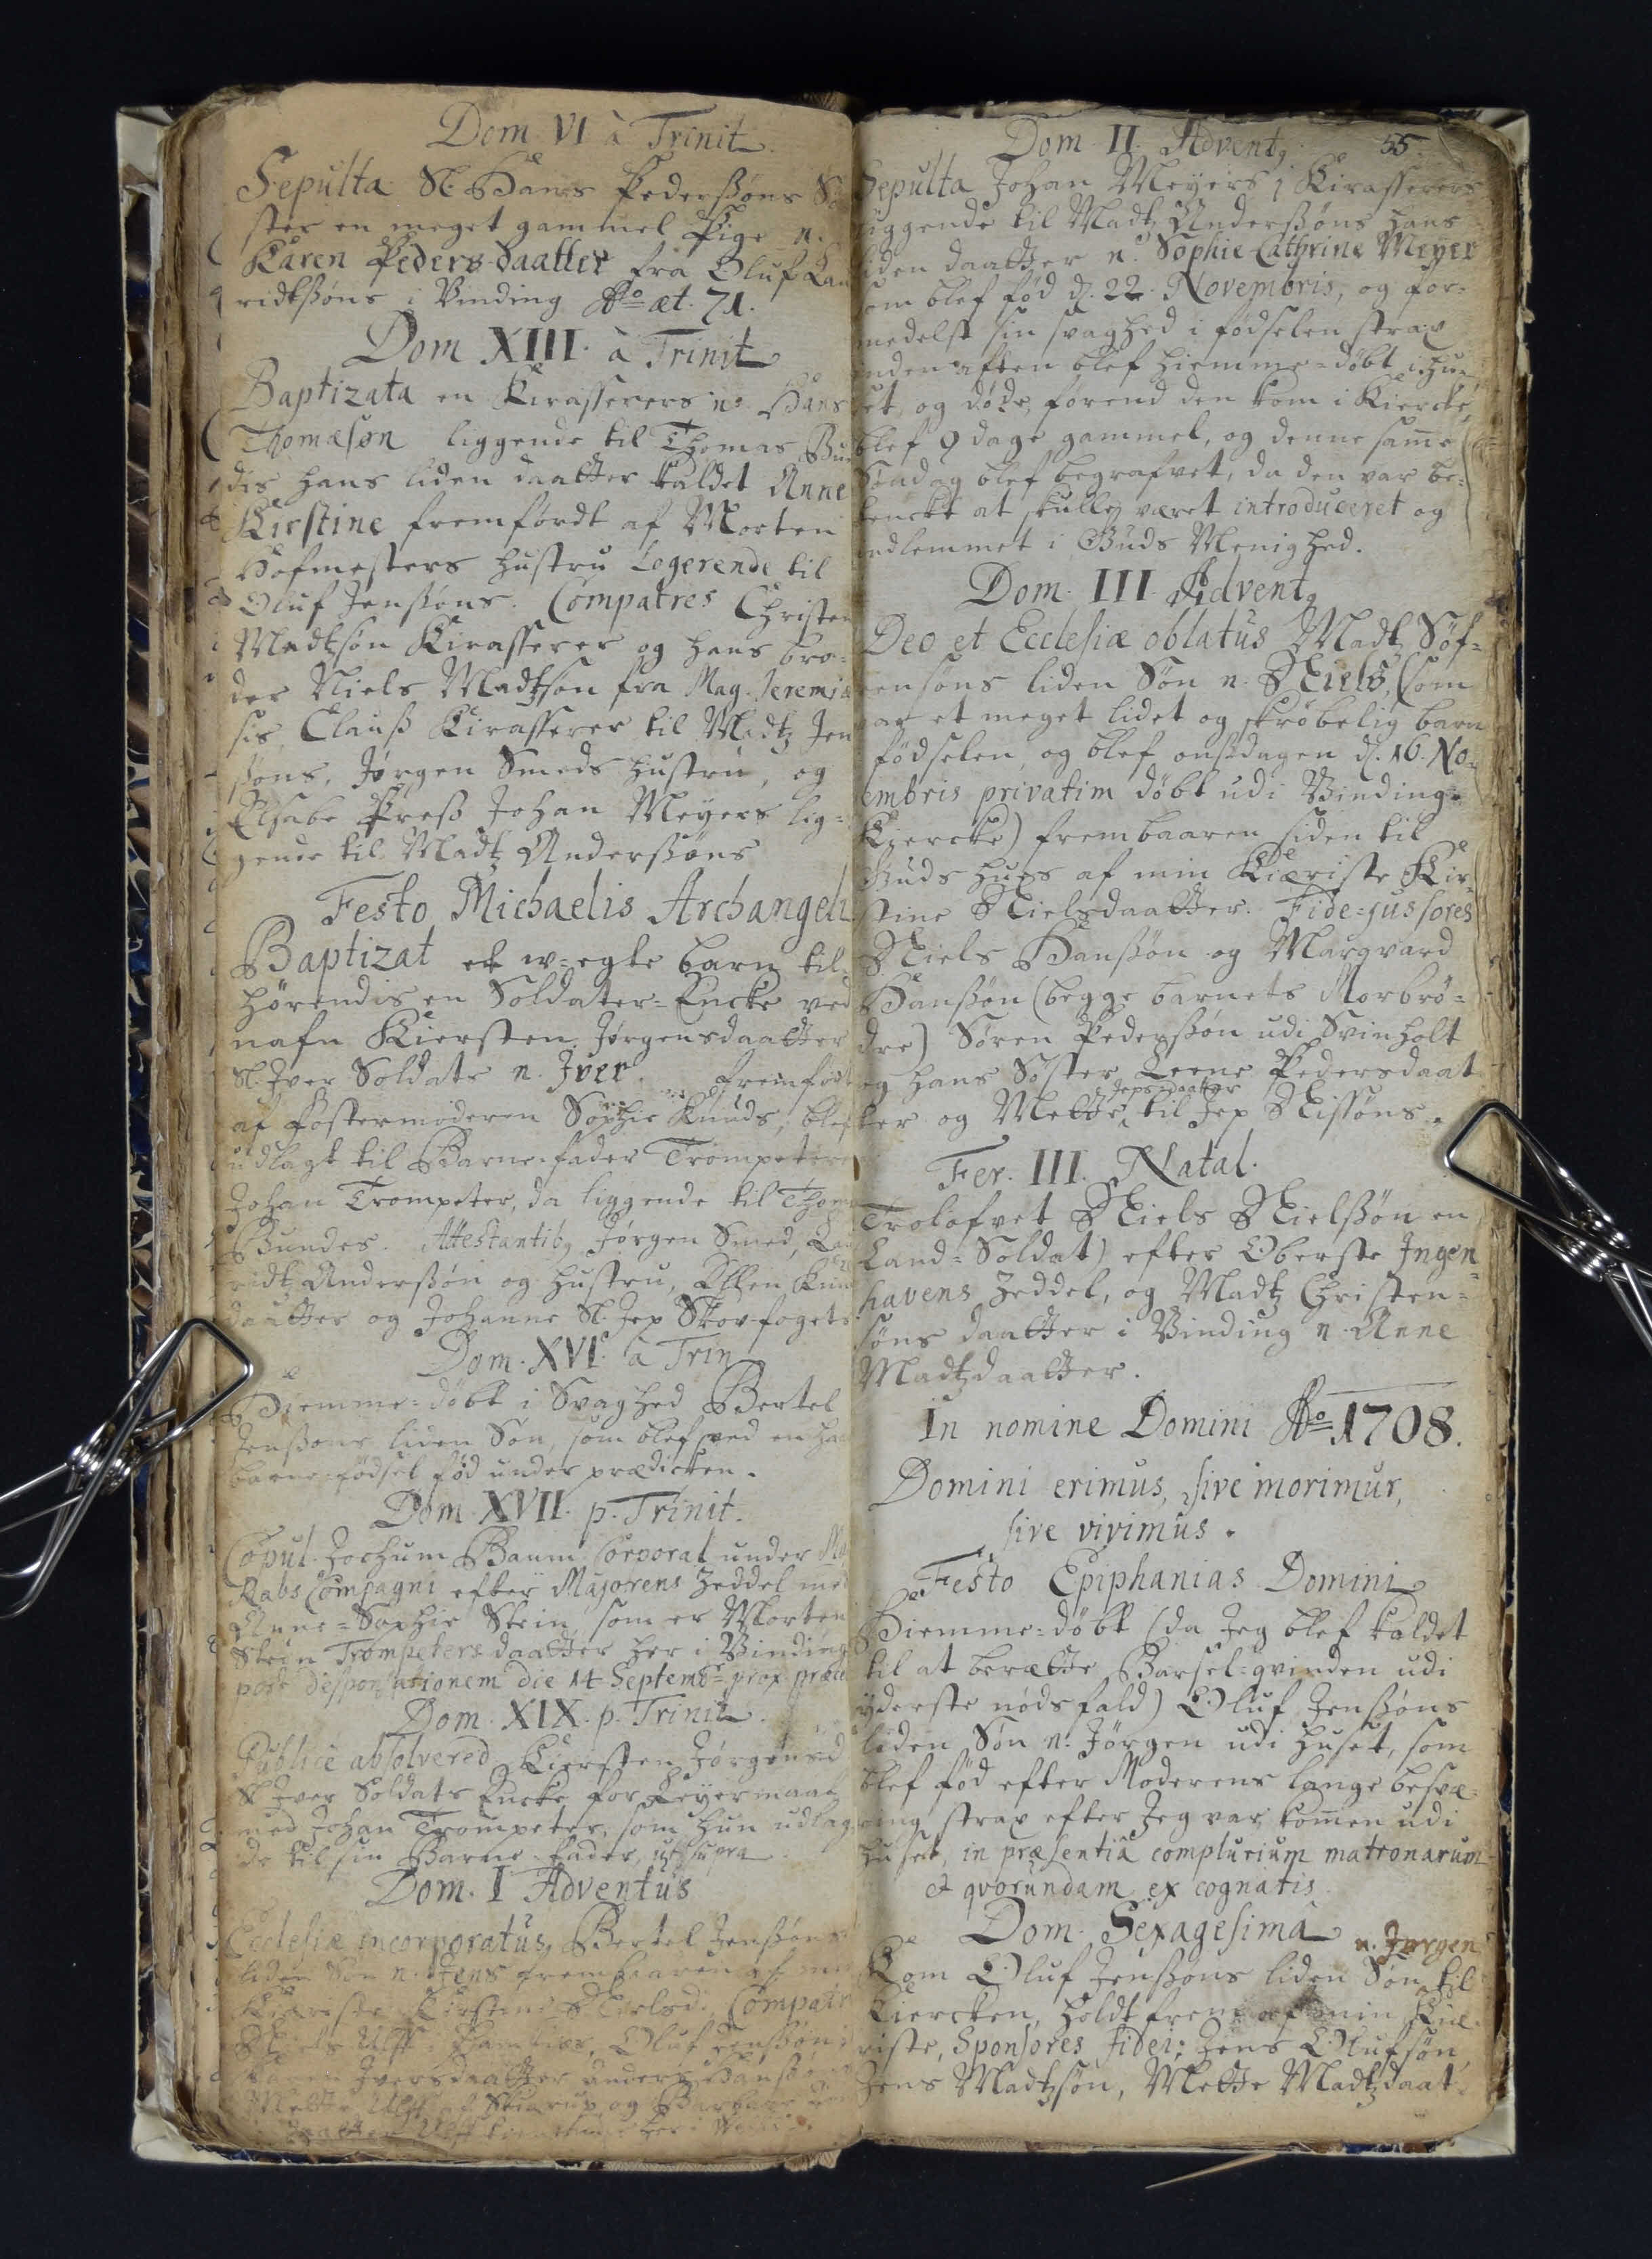Dom. VI a Trinit
Sepulta. Sl. Hans Pederssøns Sø
ster en meget gammel Pige n.
Karen Peders-daatter fra Oluf Lau
ridtssøns i Vinding Ao æt. 71.
Dom XIII. a Trinit
Baptizata en Kirasserer n. Hans
Thomæsøn liggende til Thomas Bun
dis hans liden daatter kaldet Anne
Kirstine fremførdt af Morten
Hofmesters hustru Logerende til
Oluf Jenssøns. Compatres Christen
Madtsøn Kirasserer og hans bro¬
der Niels Madtsøn fra Mag. Jeremiæ
sis, Claus Kirasserer til Madtz Jen
ssøns, Jørgen Smeds hustru, og
Elsabe Press Johan Meyers lig¬
gende til Madtz Anderssøns
Festo Michaelis Archangetz.
Baptizat et w=egte barn til
hørendis en Soldater=Encke ved
nafn Kiersten Jørgensdaatter
Sl. Iver Soldats n. Iver, fremført
af Fostermoderen Sophie Knuds, blef
udlagt til Barne=fader Trompeter
Johan Trompeter, da liggende til
Bundes. Attestantibq Jørgen Smed, Lau
ridtz Anderssøn og hustru, Ellen
daatter og Johanne Sl. Jep Skov-fogets
Dom. XVI a. Trin
Hiemme = døbt i Svaghed Bertel
Jenssøns liden Søn, som blef ved
barne = fødsel fød under prædicken.
Dom. XVII. p. Trinit.
Copul. Jochum Baum Corporal under Ma 
Rabs Compagni efter Majorens Zeddel med
Anne = Sophie Stein, som er Morten
Stein Trompeters daatter her i Vinding
post## Desponsationem die 14 Septemb = prox## præ##
Dom. XIX. p. Trinit.
Publice absolvered Kiersten Jørgensd
Sl. Ivers Soldats Encke for Leyermaal
med Johan Trompeter, som hun udlag¬
de til sin Barne = fader, ut supra.
Dom. I Adventus
Ecclesiæ in corporatus Bertel Jenssøns
liden Søn n. Jens frembaaren af
Kiæriste Kierstine Nielsd. Compatr 
Niels Ulff i Damkier. Oluf Jenssøn.
Iversdaatter Anders Hanssøns
Mette Ulff af Skiærup og Barbara
datter Ulff ## hos i W##
55
Dom. II. Adventq
Sepulta Jørgen Meyers 1 Kirasserers
liggende til Madtz Anderssøns hans
liden daatter n. Sophie Cathrine Meyer
som blef fød d. 22 Novembris, og for¬
medelst sin svaghed i fødselen strax
inden aften blef hiemme=døbt i hu¬
set, og døde førend den kom i Kircke,
blef 9 dage gammel, og denne samme
Søndag blef begrafvet, da den var be¬
tenckt at skulle været introduceret og
indlemmet i Guds Menighed.
Dom. III Adventq
Deo et Ecclesiæ oblatus Madtz Søf¬
rensøns liden Søn n. Niels (som
var et meget lidet og skrøbelig barn
fødselen, og blef onssdagen d. 16 No¬
embris privatim døbt udi Vinding
Kircke) frembaaren siden til
Guds Huus af min Kiæriste Kir¬
stine Nielsdaatter. Fide=jussores
Niels Hanssøn og Marqvard
Hanssøn (begge barnets Morbrø¬
dre) Søren Pederssøn udi Svinholt
og hans Søster Leene Pedersdaat¬
Jepsdaatter
ter og Mette til Jep Nissøns.
Fer. III. Natal.
Trolofvet Niels Nielssøn en
Land = Soldat efter Oberste Ingen¬
havens Zeddel, og Madtz Christen¬
søns daatter i Vinding n. Anne
Madtzdaatter.
In nomune Domini Ao 1708.
Domini erimus, sive morimur,
sive vivimus.
Festo Epiphanias Domini
Hiemme=døbt (da jeg blef kaldet
til at berætte Barsel=qvinden udi
yderste nøds fald) Oluf Jenssøns
liden Søn n. Jørgen udi huset, som
blef fød efter Moderens lange besvæ¬
ring strax efter jeg var komen udi
huset, in præsentia complurium matronarum
et qvorundam ex cognatis
Kom Oluf Jenssøns liden Søn til
Dom. Sexagesima n. Jørgen
n. Jørgen
Kircken, holdt frem af min Kiæ¬
riste. Spopnsores fidei; Jens Olufsøn,
Jens Madtzsøn, Mette Madtzdaat¬
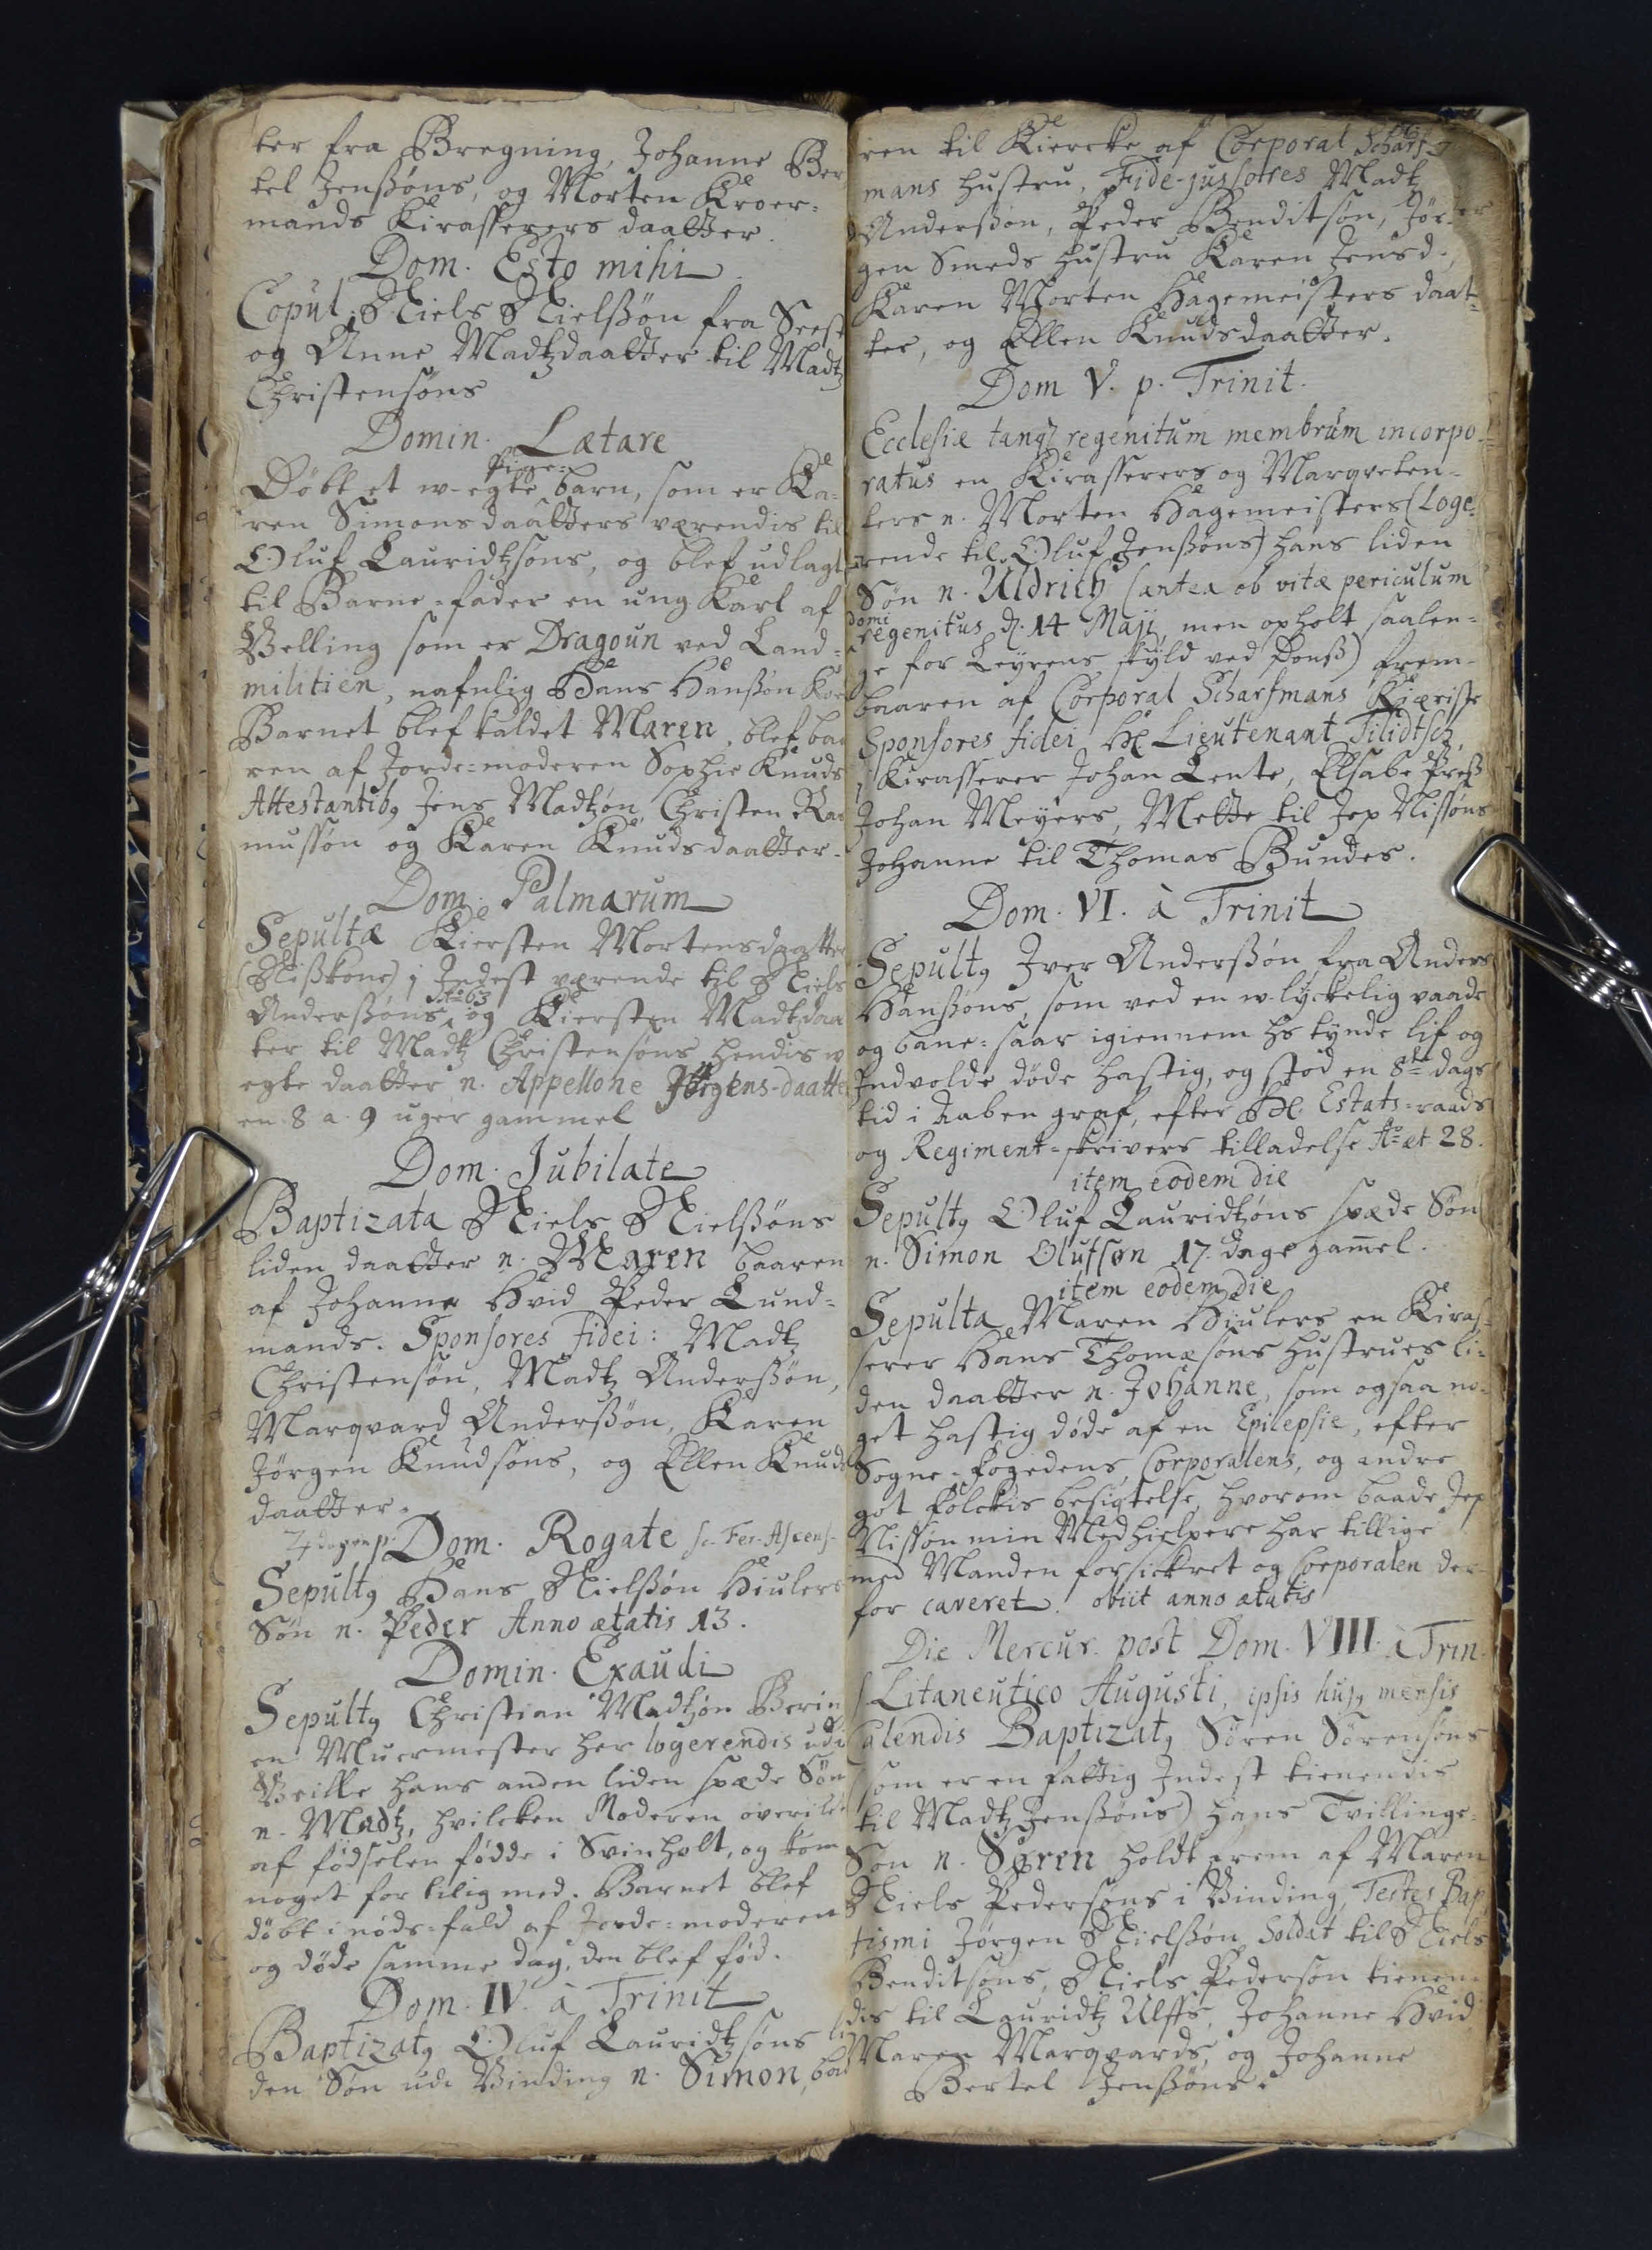ter fra Breining, Johanne Ber¬
tel Jenssøns, og Morten Kroer¬
mands Kirasserers daatter
Dom. Esto mihi
Copul. Niels Nielssøn fra Seest
og Anne Madtzdaatter til Madtz
Christensøns
Domin. Lætare
Pige=
Døbt et w=egte barn, som er Ka¬
ren Simonsdaatters værendis til
Oluf Lauridtzsøns, og blef udlagt
til Barne=fader en ung Karl af
Velling som er Dragoun ved Land¬
militien, nafnlig Hans Hanssøn
Barnet blef kaldet Maren, blef baa
ren af Jorde=moderen Sophie Knuds
Attestantibq Jens Madtzsøn, Christen Ras
mussøn og Karen Knudsdaatter.
Dom. Palmarum
Sepultæ Kirsten Mortensdaatter
(Niss Kone), 1 Inderst værende til Niels
Ao 63
Anderssøns og Kiersten Madtzdaa
ter til Madtz Christensøns hendis w
egte daatter n. Appellone Jørgens-daatter
en 8 a 9 uger gammel
Dom. Jubilate
Baptizata Niels Nielssøns
liden daatter n. Maren, baaren
af Johanne Hvid Peder Lund¬
mands. Sponsores fidei: Madtz
Christensøn, Madtz Anderssøn,
Marqvard Anderssøn. Karen
Jørgen Knudsøns, og Ellen Knuds
daatter.
dagen p. Dom. Rogate sc. Fer. Ascens.
Sepultq Hans Nielssøn Hiulers
Søn n. Peder Anno ætatis 13.
Domin. Exaudi
Sepultq Christian Madtzøn Berin
en Muermester her logerendis udi
Veille hans anden liden spæde Søn
n. Madtz, hvilken Moderen
af fødselen fødde i Svinholt, og kom
nogen for tilig med. Barnet blef
døbt i nøds=fald af Jorde=moderen
og døde samme dag, den blef fød.
Dom. IV. a Trinit
Baptizatq Oluf Lauridtzøns li
den Søn udi Vinding n. Simon ba 
ren til Kiercke af Corporal Scharf
mans hustru, Fide-jussores Madtz
Anderssøn, Peder Benditsøn, Jør¬
gen Smeds hustru Karen Jensd.
Karen Morten Hagemeisters daat¬
ter, og Ellen Knudsdaatter.
Dom V. p. Trin
Ecclesie tanq## regenitum membrum incorpo¬
ratus en Kirasserers og Marqveten¬
ters n. Morten Hagemeisters (loge¬
rende til Oluf Jenssøns) hans liden
Søn n. Uldrich (antea ob vitæ periculum 
domi
regenitus d. 14 Maji, men opholt saalen¬
ge for Leyrens skyld ved Donss) frem¬
baaren af Corporal Scharfmans Kiæriste
Sponsores fidei Hl Lieutenant Tilidtsch
Kirasserer Johan Lente, Elsabe Press
Johan Meyers, Mette til Jep Nissøns
Johanne til Thomas Bundes.
Dom. VI. a Trin
Sepultq Iver Anderssøn fra Anders
Hanssøns, som ved en w=lyckelig vaade
og bane=saar igiennem hs tynde lif og
Indvolde døde hastig, og stod en 8te dags
tid i Aaben graf, efter Hl Estats=raads
og Regiment=skrivers tilladelse Ao æt 28.
item eodem die
Sepultq Oluf Lauridtzøns spæde Søn
n. Simon Olufsøn 17. dage gammel
item eodem die
Sepulta Maren Hiulers en Kiras¬
serer Hans Thomæsøns hustrues li¬
den daatter n. Johanne, som ogsaa no¬
get hastig døde af en Epilepsie, efter
Sogne - Fogedens, Corporalens, og andre
got Folckis besigtelse, hvorom baade Jep
Nissøn min Medhielpere har tillige
med Manden forsickret og Corporalen der¬
for caveret. obiit anno ætatis
Die Mercur post Dom. VIII a Trin.
Litaneutico Augusti, ipsis husq mersis
Calendis Baptizatq Søren Sørensøns
som er en fattig Inderst tienendis
til Madtz Jenssøns) hans Tvillinge¬
Søn n. Søren holdt frem af Maren
Niels Pedersøns i Vinding. Testes Bap
tismi Jørgen Nielssøn Soldat til Niels
Benditsøns, Niels Pedersøn tienen¬
dis til Lauridtz Ulffs, Johanne Hvid
Maren Marqvards, og Johanne 
Bertel Jenssøns.